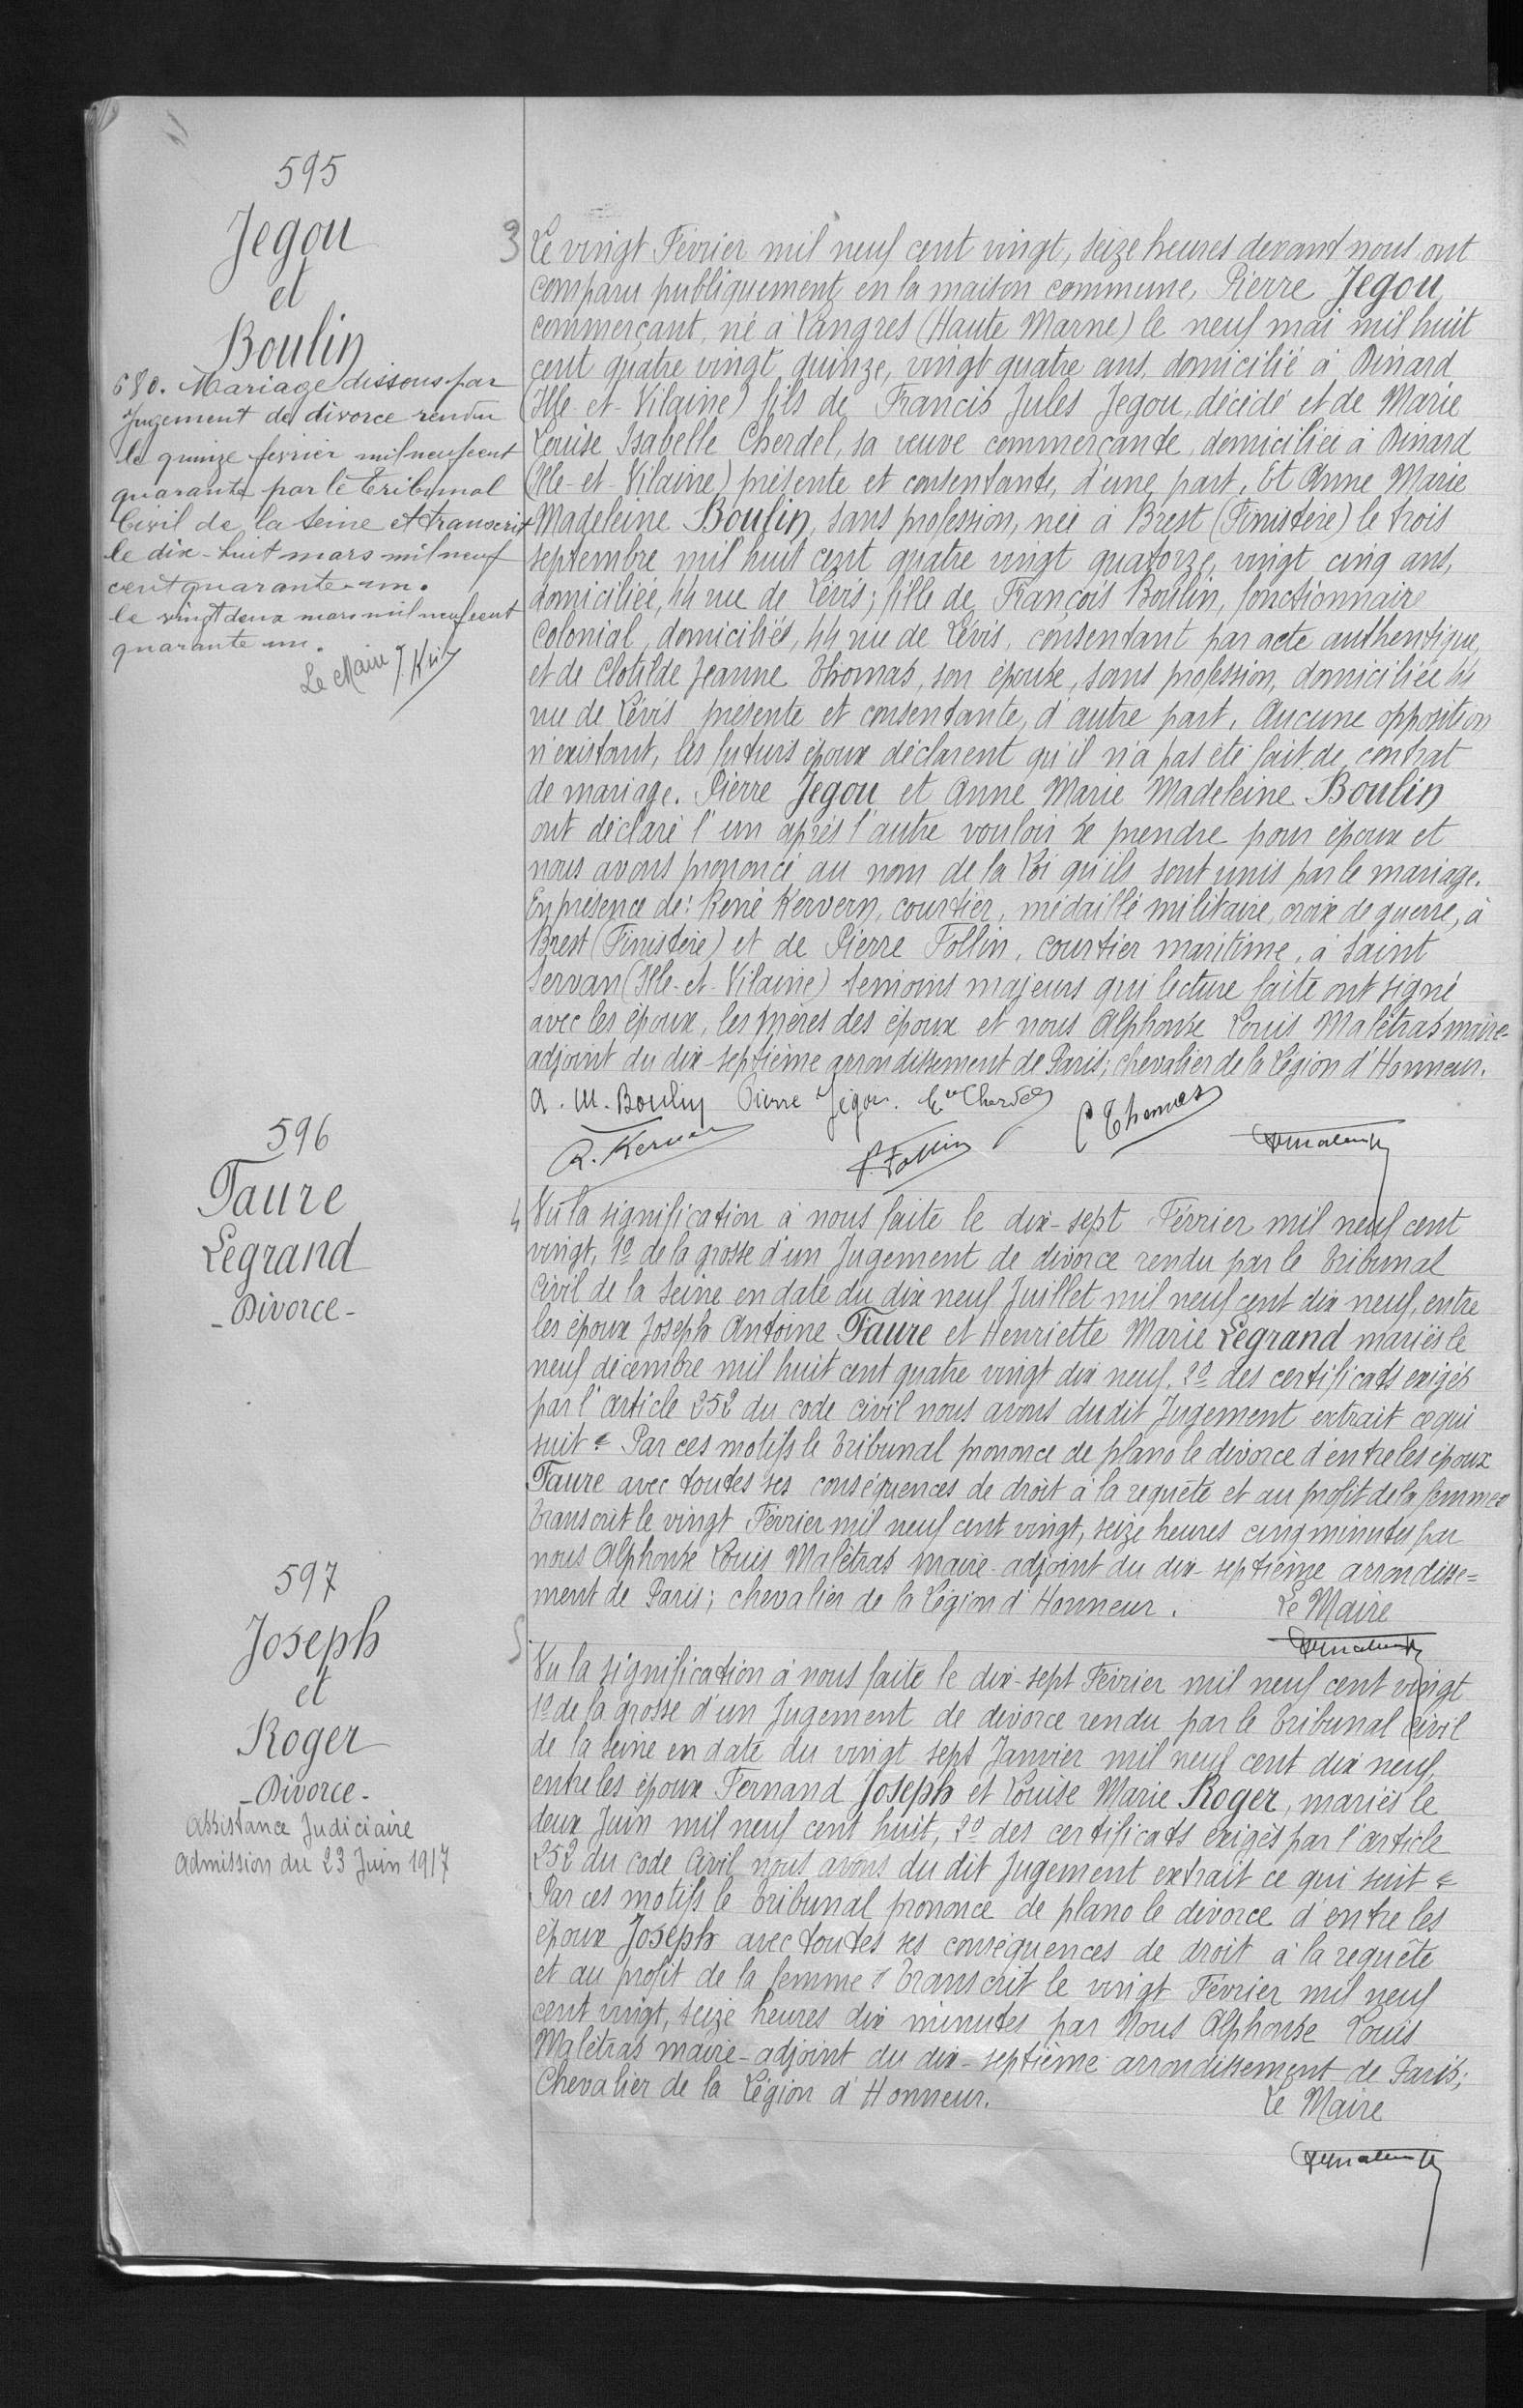595
Jegou
et
Boulin
Le vingt Février mil neuf cent vingt, seize heures devant nous ont
comparu publiquement en la maison commune, Pierre Jegou
commerçant, né à Vangres (Haute Marne) le neuf mai mil huit
cent quatre vingt quinze, vingt quatre ans, domicilié à Dinard
(Ile-et-Vilaine) fils de Francis Jules Jegou, décédé et de Marie
Louise Isabelle Cherdel, sa veuve commerçante domiciliée à Dinard
(Ile-et-Vilaine) présente et consentante, d'une part, et Anne Marie
Madelein Boulin, sans profession, née à Brest (Finistère) le trois
septembre mil huit cent quatre vingt quatorize, vingt cinq ans,
domiciliée, 44 rue de Lévis; fille de François Boulin, fonctionnaire
colonial domiciliés, 44 rue de Lévis, consentant par acte authentique,
et de Clotilde Jeanne Thomas, son épouse, sans profession, domiciliée 44
rue de Lévis présente et consentante, d'autre part, Aucune opposition
n'existant, les futurs époux déclarent qu'il n'a pas été fait de contrat
de mariage. Pierre Jegou et Anne Marie Madelein Boulin
ont déclaré l'un après l'autre vouloir se prendre pour époux et
nous avons prononcé au nom de la loi qu'ils sont unis par le mariage.
En présence de: René Revern, courtier, médaillé militaire, croix de guerre, à
Brest (Finistère) et de Pierre Follin, courtier maritime, à Saint
Servan (Ille-et-Vilaine) témoins majeurs qui lecture faite ont signé
avec les époux, les mères des époux et nous Alphonse Louis Malètre maire-
adjoint du dix-septième arrondissement de Paris; Chevalier de la légion d'Honneur.
596
Faure
Legrand
-Divorce-
597
Joseph
et
Roger
-Divorce-
Vu la signification à nous faite le dix-sept Février mil neuf cent
vingt 1° de la grosse d'un Jugement de divorce rendu par le Tribunal
Civil de la Seine en date du dix neuf Juillet mil neuf cent dix-neuf, entre
les époux Joseph Antoine Faure et Henriette Marie Legrand mariés le
neuf décembre mil huit cent quatre vingt dix neuf 2° du certificats exigés
par l'article 252 du code civil nous avons dudit Jugement extrait ce qui
suit: Par ces motifs les Tribunal prononce de plano le divorce d'entre les époux
Faure avec toutes ses conséquences de droit à la requête et au profit de la femme
transcrit le vingt Février mil neuf cent vingt, seize heures cinq minutes par
nous Alphonse Louis Malètras Maire-adjoint du dix-septième arrondisse-
ment de Paris; Chevalier de la Légion d'Honneur. Le Maire
Vu la signification à nous faite le dix-sept Février mil neuf cent vingt
1° de la grosse d'un Jugement de divorce rendu par le Tribunal civil
de la Seine en date du vingt sept Janvier mil neuf cent dix neuf
entre les époux Fernand Joseph et Louise Marie Roger, mariés le
deux Juin mil neuf cent huit, 2° des certificats exigés par l'article
252 du Code Civil nous avons du dit Jugement extrait ce qui suit:
Par ces motifs le Tribunal prononce de plano le divorce d'entre les
époux Joseph avec toutes ses conséquences de droit à la requête
et aur profit de la femme; Transcrit le vingt Fevrier mil neuf
cent vingt, seize heures dix minutes par Nous Alphonse Louis
Malètras maire-adjoint du dix-septième arrondissement de Paris,
Chevalier de la Légion d'Honneur. Le Maire
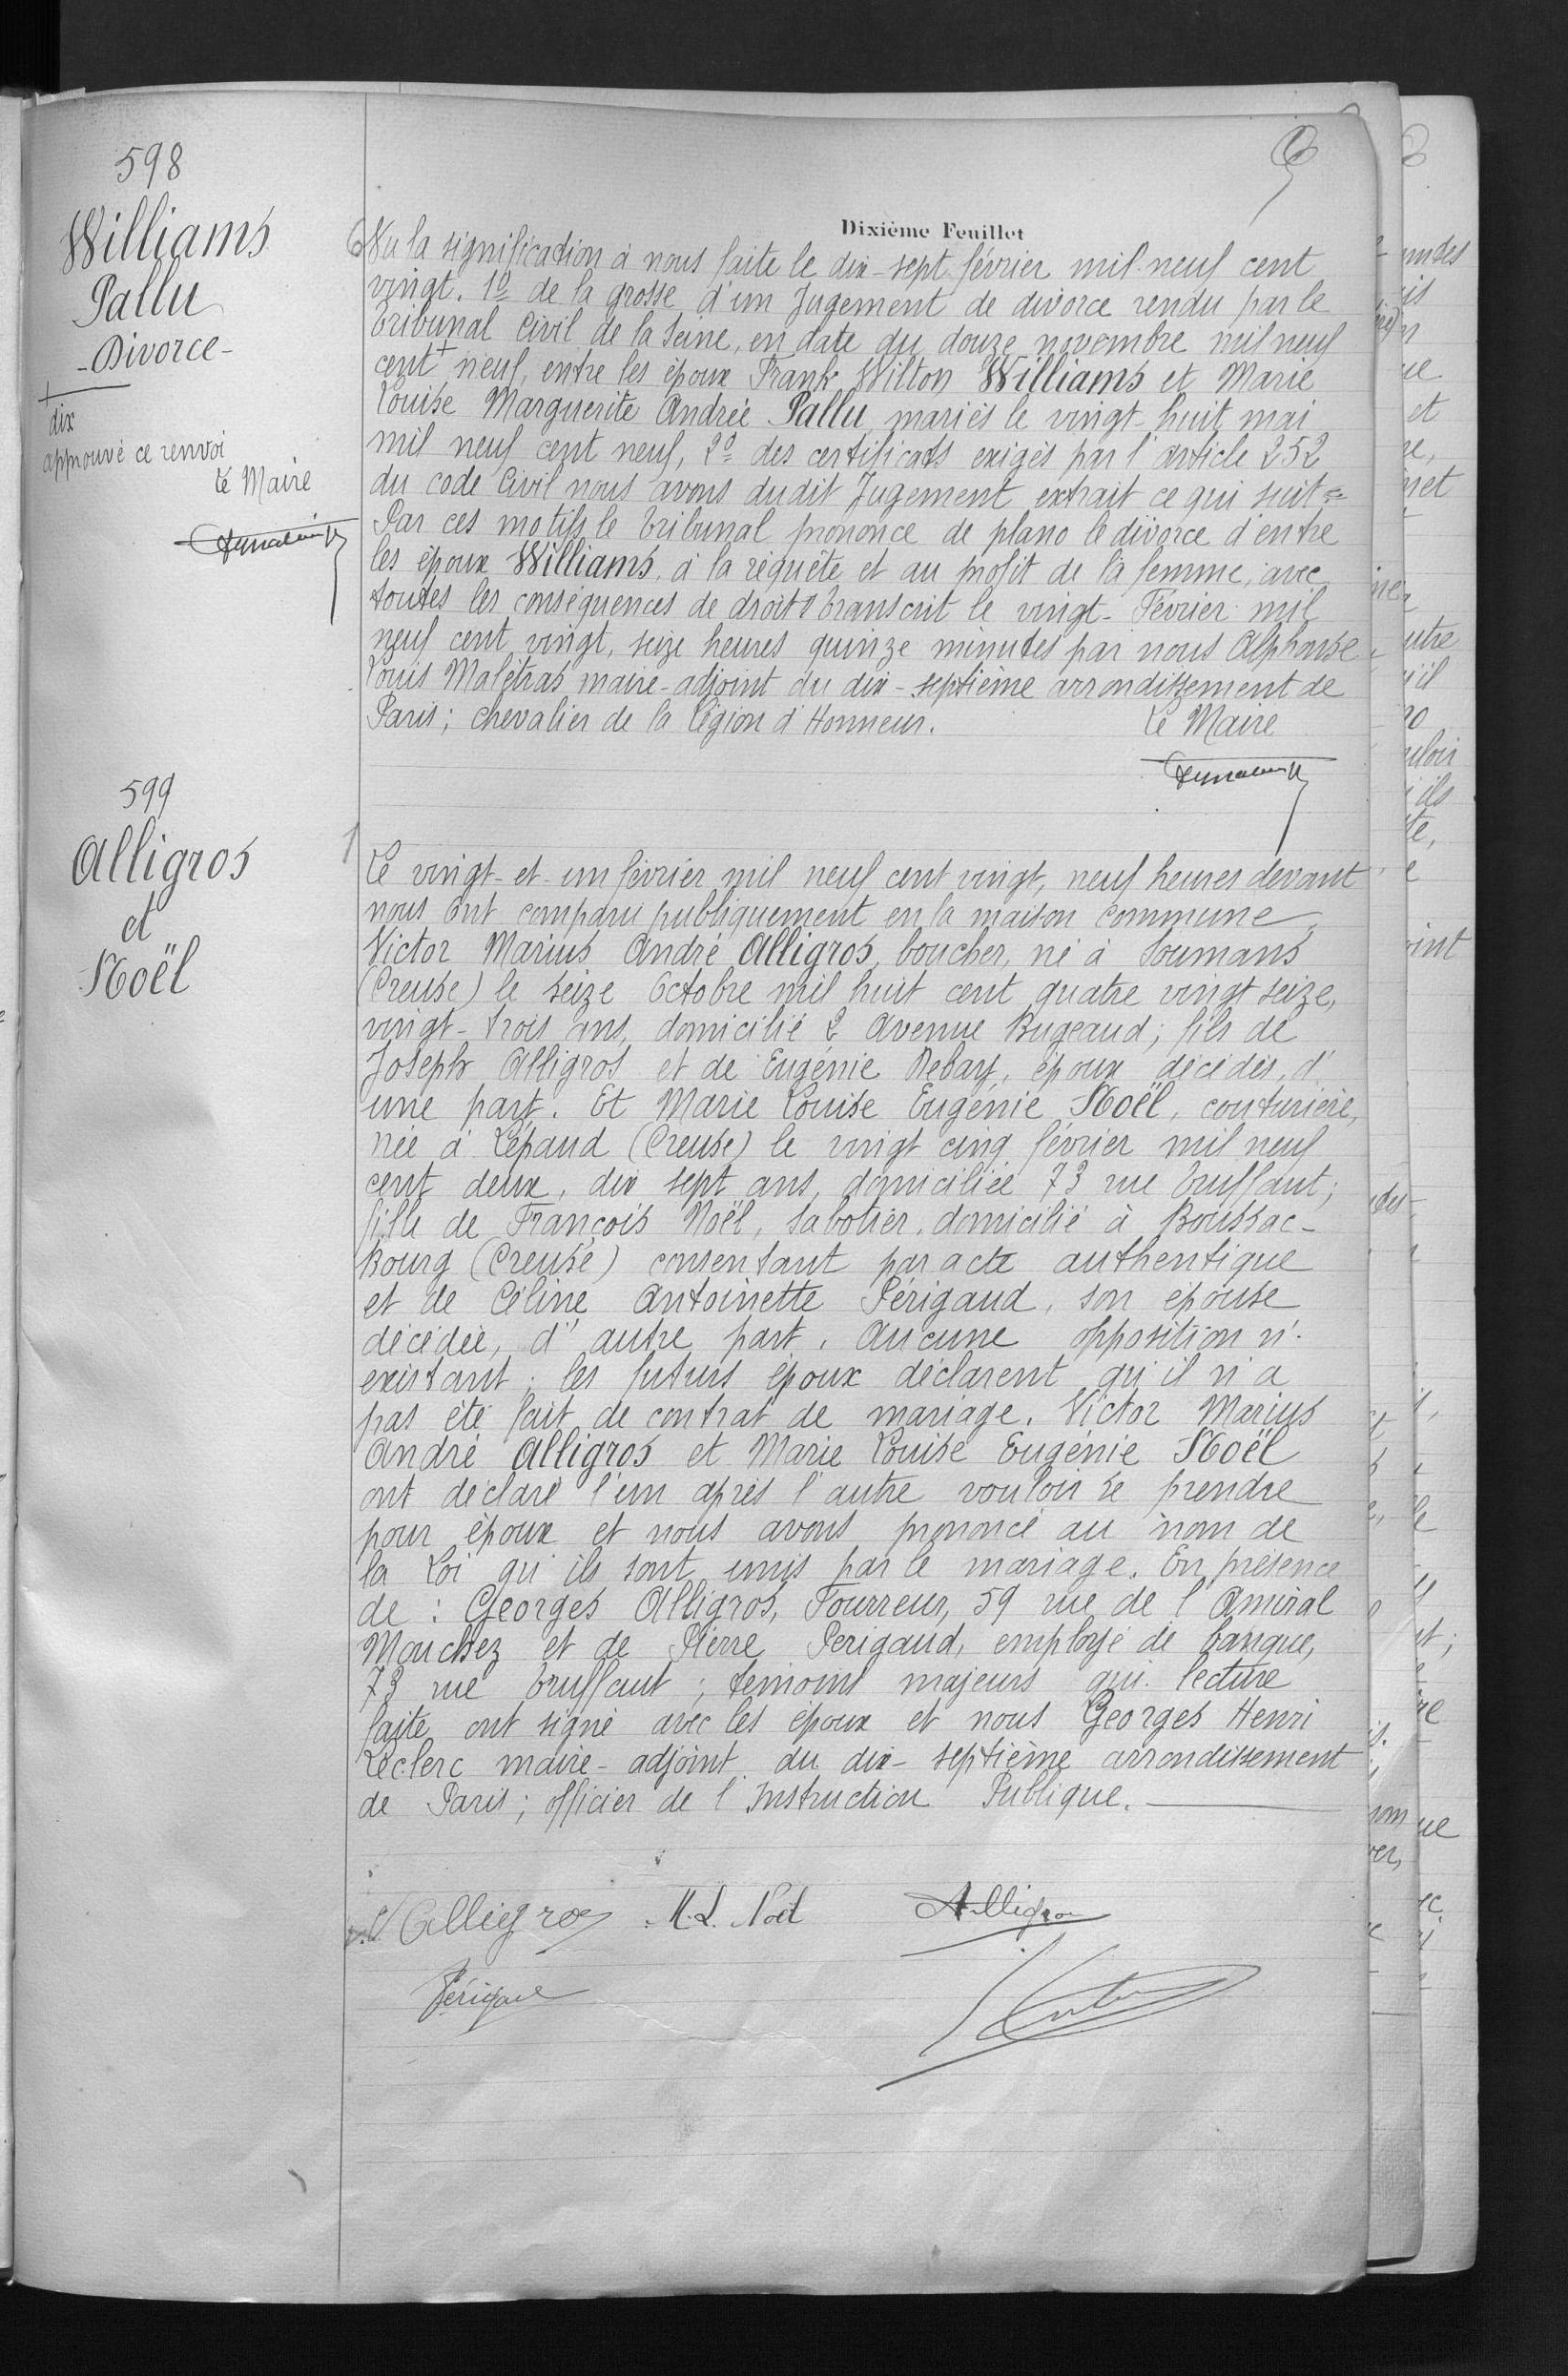598
Williams
Pallu
599
Alligros
et Noël
Vu la signification à nous faite le dix-sept février mil neuf cent
vingt 1° de la grosse d'un Jugement de divorce rendu par le
Tribunal Civil de la Seine en date du douze novembre mil neuf
cent neuf entre les époux Frank Wilton Williams et Marie
Louise Marguerite Andrée Pallu mariés le vingt-huit mai
mil neuf cent neuf, 2° des certificats exigés par l'article 252
du code Civil nous avons du dit Jugement extrait ce qui suit:
Par ces motifs le Tribunal prononce de plano le divorce d'entre
les époux Williams à la requête et au profit de la femme avec
toutes les conséquences de droit transcrit le vingt Février mil
neuf cent vingt, seize heures quinze minutes par nous Alphonse
Louis Malétras mair-adjoint du dix-septième arrondissement de
Paris; Chevalier de la légion d'Honneur. Le Maire.
Le vingt-et-un février mil neuf cent vingt, neuf heure devant
nous ont comparu publiquement en la maison commune
Victor Marius, André Alligros boucher, né à Soumans
(Creuse) le seize Octobre mil huit cent quatre vingt seize,
vingt-trois ans, domicilié 2 avenue Bugeaud; fils de
Joseph Alligros et de Eugénie Debay, époux décédés, d'
une part. Et Marie Louise Eugénie Noël, couturière
née à Lépaud (Creuse) le vingt cinq février mil neuf
cent deux, dix sept ans, domiciliée 73 rue Truffaut;
fille de François Noël, sabotier, domicilié à Boussac-
Bourg (Creuse) consentant par acte authentique
et de Céline Antoinette Périgaud, son épouse
décédée, d'autre part, aucune opposition n'
existant. Les futurs époux déclarent qu'il n'a
pas été fait de contrat de mariage. Victor Marius
André Alligros et Marie Louise Eugénie Noël
ont déclaré l'un après l'autre vouloir se prendre
pour époux et nous avons prononcé au nom de
la Loi qu'ils sont unis par le mariage. En présence
de: Georges Alligros Fourreur, 59 rue de l'Amiral
Mouchez et de Pierre Perigoud, employé de banque,
73 rue Truffaut; témoins majeurs qui lecture
faite ont signé avec les époux et nous Georges Henri
Leclerc maire-adjoint du dix-septième arrondissement
de Paris; officier de l'Instruction Publique, -